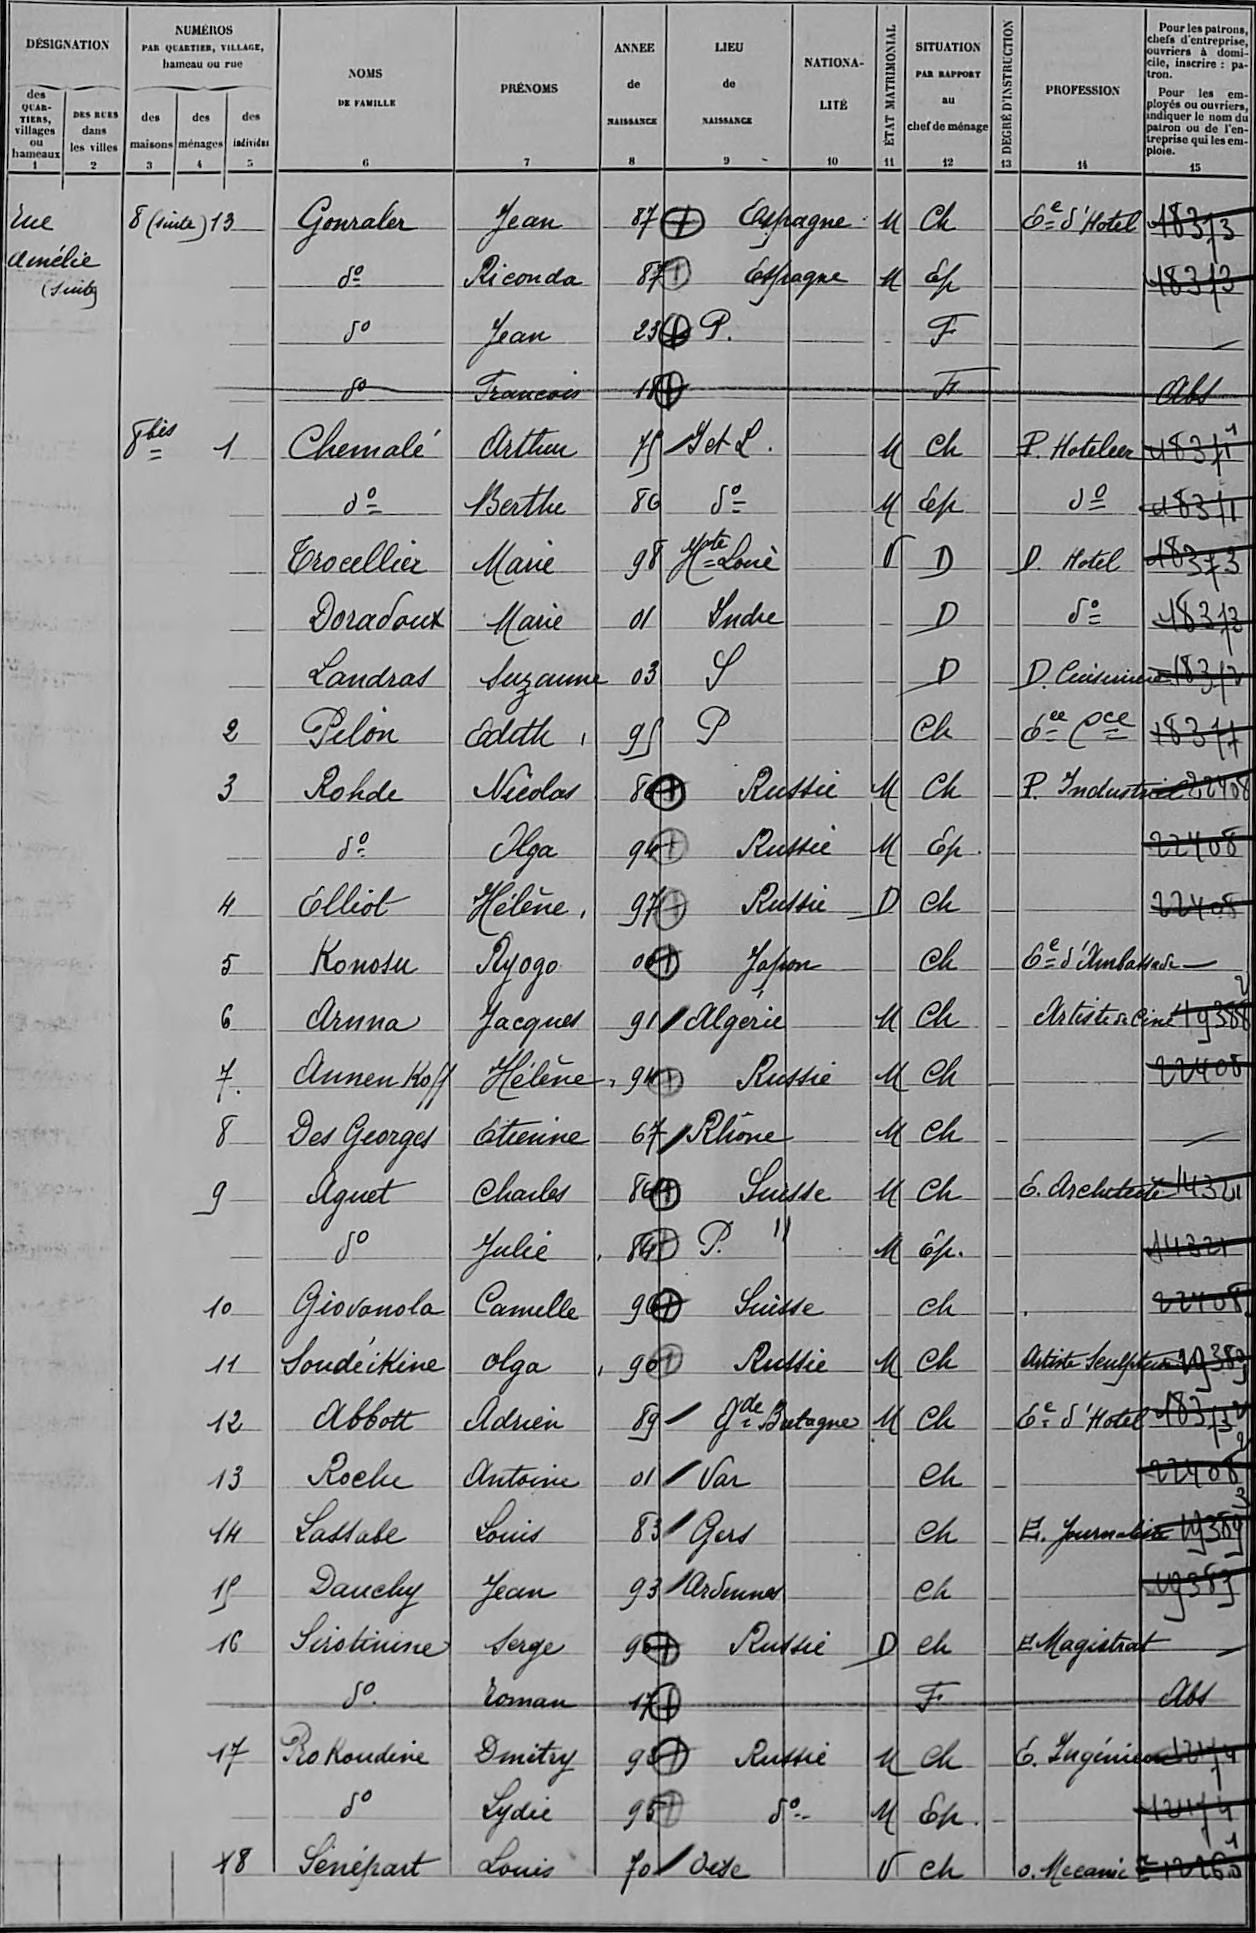Gouraler/Jean/87/Espagne/¤/M/Ch/¤/Ee dHotel/18373
d°/Riconda/87/Espagne/¤/M/Ep/¤/¤/18373
d°/Jean/23/P /¤/¤/F/¤/¤/¤
d°/François/18/¤/¤/¤/F/¤/¤/abs
Chemalé/Arthur/75/I et L /¤/M/Ch/¤/P Hotelier/18371
d°/Berthe/86/d°/¤/M/Ep/¤/d°/18371
Trocellier/Marie/98/Hte Loire/¤/V/D/¤/D Hotel/18373
Doradoux/Marie/01/Indre/¤/¤/D/¤/d°/18373
Landras/Suzanne/03/S/¤/¤/D/¤/D Cuisinière/18372
Pilon/Edith/95/P/¤/¤/Ch/¤/Eee Cce/18377
Rohde/Nicolas/86/Russie/¤/M/Ch/¤/P Industrie/22408
d°/Ilga/94/Russie/¤/M/Ep /¤/¤/22408
Elliot/Hélène/97/Russie/¤/D/Ch/¤/¤/22408
Konosu/Ryogo/00/Japon/¤/¤/Ch/¤/Ee d'Ambassade/¤
Aruna/Jacques/91/Algérie/¤/M/Ch/¤/Artiste de ciné/19388
Aunen koff/Hélène/94/Russie/¤/M/Ch/¤/¤/22408
Des Georges/Etienne/67/Thône/¤/M/Ch/¤/¤/¤
Aguet/Charles/86/Suisse/¤/M/Ch/¤/E Architecte/14321
d°/Julie/84/P /¤/M/Ep /¤/¤/14321
Giovanola/Camille/96/Russie/¤/M/Ch/¤/¤/22408
Soudéikine/olga/90/Russie/¤/M/Ch/¤/Artiste Sculpteur/19389
Abbott/Adrien/89/Gde Bretagne/¤/M/Ch/¤/Ee d'Hotel/18373
Roche/Antoine/01/Var/¤/¤/Ch/¤/¤/22408
Lassabe/louis/83/Gers/¤/¤/Ch/¤/E Journaliste/19389
Dauchy/Jean/93/Ardennes/¤/¤/Ch/¤/¤/19389
Sirstinine/Serge/96/Russie/¤/D/Ch/¤/E Magistrat/¤
d°/Roman/17/¤/¤/¤/F/¤/¤/abs
Rokoudine/Dmitry/93/Russie/¤/M/Ch/¤/E Ingénieur/12174
d°/Lydie/95/d°/¤/M/Ep /¤/¤/12174
Sénépart/Louis/70/Oise/¤/V/Ch/¤/o Mécanic/12260
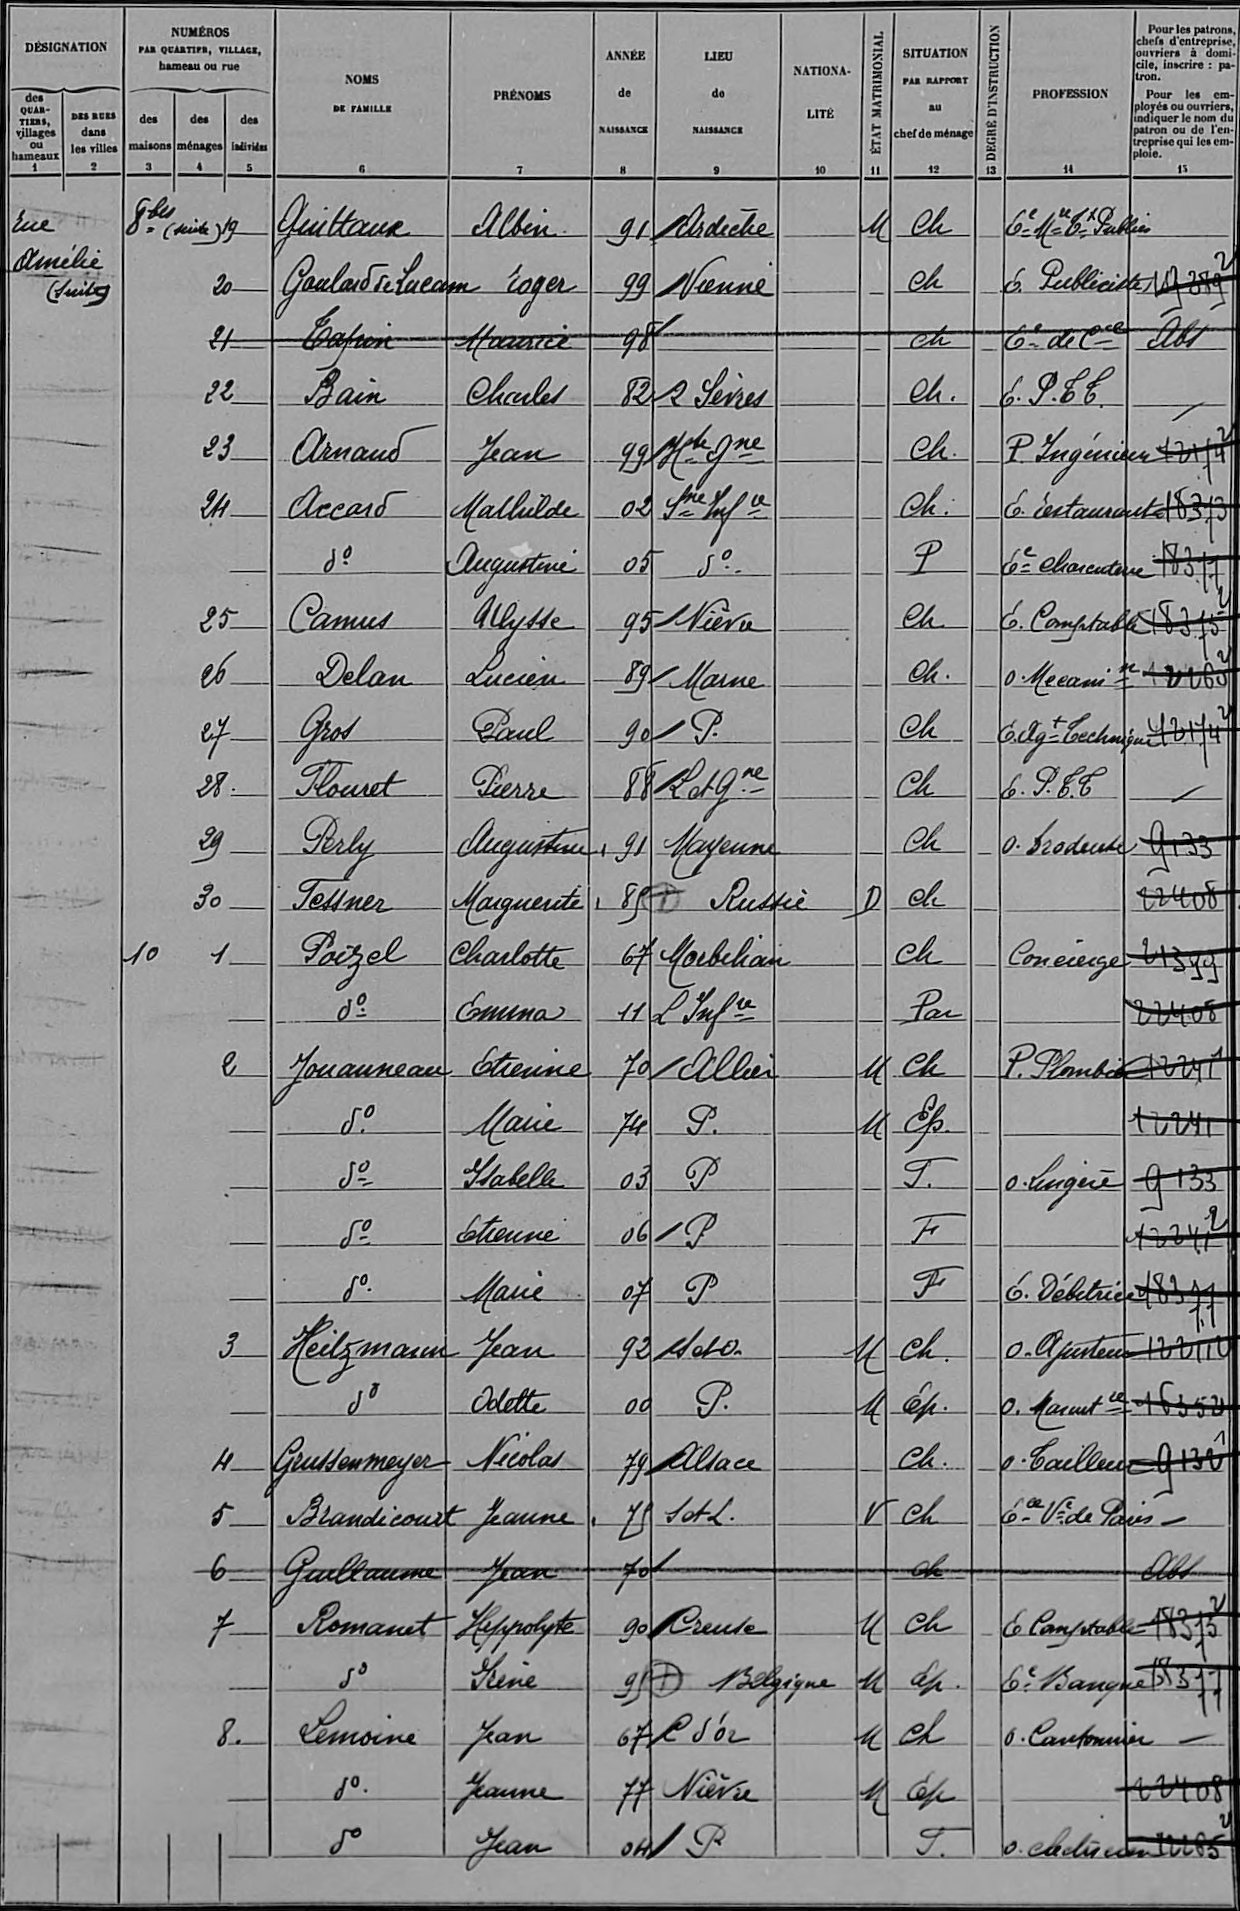Guittaur/Albin/91/Ardèche/¤/M/Ch/¤/Ee Mre Tt Publics/¤
Gaulard de Lucam/Roger/99/Vienne/¤/¤/Ch/¤/E Publiciste/19389
Tapin/Maurice/98/¤/¤/¤/ch/¤/Ee de Cce/abs
Bain/Charles/82/2 Sèvres/¤/¤/Ch /¤/E P T T /¤
Arnaud/Jean/99/Hte Gne/¤/¤/Ch /¤/P Ingénieur/12174
Accard/Mathilde/02/Sne Infre/¤/¤/Ch /¤/E restaurant/18373
d°/Augustine/05/d°/¤/¤/P/¤/Ee charcuterie/18377
Camus/Ulysse/95/Nièvre/¤/¤/Ch/¤/E Comptable/18375
Delan/Lucien/89/Marne/¤/¤/Ch /¤/O Mécanin/12260
Gros/Paul/90/P /¤/¤/Ch/¤/E Agt Technique/12174
Flouet/Pierre/88/L et Gne/¤/¤/Ch/¤/E P T T /¤
Perly/Augustine/91/Mayenne/¤/¤/Ch/¤/o brodeuse/9133
Fessner/Marguerite/85/Russie/¤/D/Ch/¤/¤/22408
Poizel/Charlotte/67/Morbihan/¤/¤/Ch/¤/Concierge/21399
d°/Emma/11/L Infre/¤/¤/Par/¤/¤/22408
Jouanneau/Etienne/70/Allier/¤/M/Ch/¤/P Plombier/12241
d°/Marie/74/P /¤/M/Ep/¤/¤/12241
d°/Isabelle/03/P/¤/¤/F /¤/o lingère/9133
d°/Etienne/06/P/¤/¤/F/¤/¤/12241
d°/Marie/07/P/¤/¤/F/¤/E Débitrice/18377
Heitzmann/Jean/92/S et O /¤/M/Ch /¤/O Ajusteur/12211
d°/Odette/00/P /¤/M/Ep /¤/o Manutre/16352
Grussenmeyer/Nicolas/79/Alsace/¤/¤/Ch /¤/o Tailleur/9132
Brandicourt/Jeanne/75/S et L /¤/V/Ch/¤/Eee Ve de Paris/¤
Guillaime/Jean/70/¤/¤/¤/Ch/¤/¤/abs
Romanet/Hippolyte/90/Creuse/¤/M/Ch/¤/E Comptable/18373
d°/Irène/95/Belgique/¤/M/Ep /¤/Ee Banque/18377
Lemoine/Jena/67/C d'Or/¤/M/Ch/¤/O Cantonnier/¤
d°/Jeanne/77/Nièvre/¤/M/Ep/¤/¤/22408
d°/Jean/04/P/¤/¤/F /¤/o electricien/32265